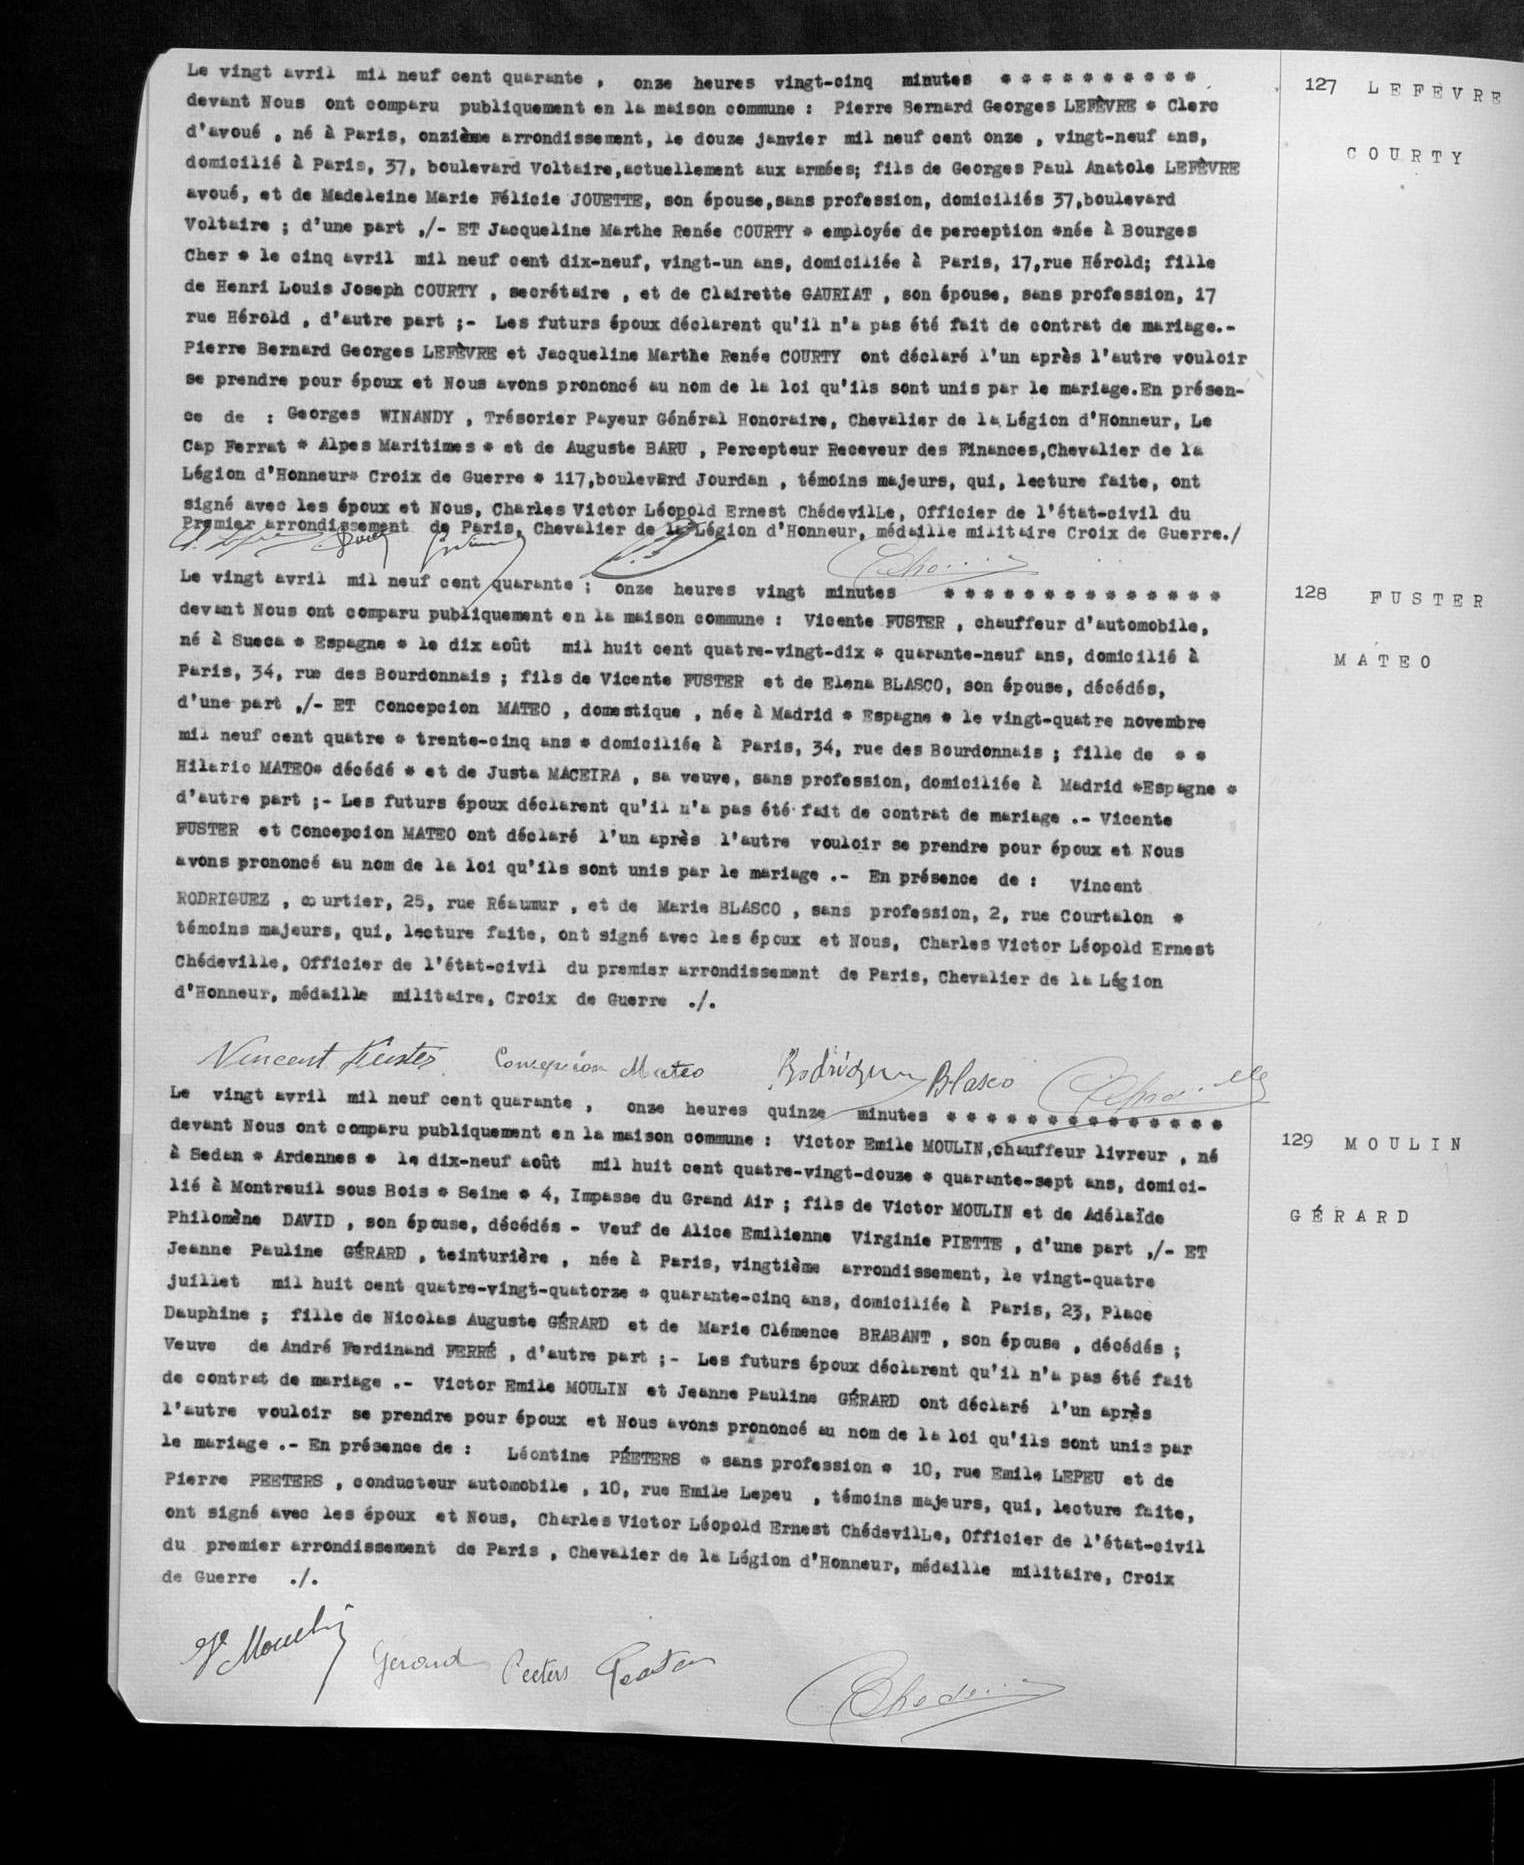Le vingt avril mil neuf cent quarante, onze heures vingt-cinq minutes ****
devant Nous ont comparu publiquement en la maison commune: Pierre Bernard George LEFEVRE * Clerc
d'avoué, né à Paris, onzième arrondissement, le douze janvier mil neuf cent onze, vingt-neuf ans,
domicilié à Paris, 37, boulevard Voltaire, actuellement aux armées; fils de Georges Paul Anatole LEFEVRE
avoué, et de Madeleine Marie Félicie JOUETTE, son épouse, sans profession, domiciliés 37, boulevard
Voltaire; d'une part ,/-ET Jacqueline Marthe Renée COURTY * employée de perception * née à Bourges
Cher * le cinq avril mil neuf cent dix-neuf, vingt-un ans, domiciliée à Paris, 17, rue Hérold; fille
de Henri Louis Joseph COURTY, secrétaire, et de Clairette GAURIAT, son épouse, sans profession, 17
rue Hérold, d'autre part ;-Les futurs époux déclarent qu'il n'a pas été fait de contrat de mariage.
Pierre Bernard Georges LEFEVRE et Jacqueline Marthe Renée COURTY ont déclaré l'un après l'autre vouloir
se prendre pour époux et Nous avons prononcé au nom de la loi qu'ils sont unis par le mariage. En présen-
ce de: Georges WINANDY, Trésorier Payeur Général Honoraire, Chevalier de la Légion d'Honneur, Le
Cap Ferrat * Alpes Maritimes * et de Auguste BARU, Pecepteur Receveur des Finances, Chevalier de la
Légion d'Honneur * Croix de Guerre * 117, boulevard Jourdan, témoins majeurs, qui, lecture faite, ont
signé avec les époux et Nous, Charles Victor Léopold Ernest Chédeville, Officier de l'état-civil du
Premier arrondissement de Paris, Chevalier de la Légion d'Honneur, médaille militaire Croix de Guerre ./
Le vingt avril mil neuf cent quarante; onze heures vingt minutes ****
devant Nous ont comparu publiquement en la maison commune: Vincente FUSTER, chauffeur d'automobile;
né à Sueca * Espagne * le dix août mil huit cent quatre-vingt-dix * quarante-neuf ans, domicilié à
Paris, 34, rue des Bourdonnais; fils de Vincente FUSTER et de Elena BLASCO, son épouse, décédés,
d'une part ,/-ET Concepcion MATEO, domestique, née à Madrid * Espagne * le vingt-quatre novembre
mil neuf cent quatre * trente-cinq ans * domiciliée à Paris, 34, rue des Bourdonnais; fille de **
Hilario MATEO * décédé * et de Juste MACEIRA, sa veuve, sans profession, domiciliée à Madrid *Espagne *
d'autre part ;-Les futurs époux déclarent qu'il n'a pas été fait de contrat de mariage .-Vincente
FUSTER et Conception MATEO ont déclaré l'un après l'autre vouloir se prendre pour époux et Nous
avons prononcé au nom de la loi qu'ils sont unis par le mariage .-En présence de: Vincent
RODRIGUEZ, courtier, 25, rue Réaumur, et de Marie BLASCO, sans profession, 2, rue Courtalon *
témoins majeurs, qui, lecture faite, ont signé avec les époux et Nous, Charles Victor Léopold Ernest
Chédeville, Officier de l'état-civil du premier arrondissement de Paris, Chevalier de la Légion
d'Honneur, médailler militaire, Croix de Guerre ./.
Le vingt avril mil neuf cent quarante, onze heures quinze minutes ****
devant Nous ont comparu publiquement en la maison commune: Victor Emile MOULIN, chauffeur livreur, né
à Sedan * Ardennes * le dix-neuf août mil huit cent quatre-vingt-douze * quarante-sept ans, domici-
lié à Montreuil sous Bois * Seine * 4, Impasse du Grand Air; fils de Victor MOULIN et de Adélaïde
Philomène DAVID, son épouse, décédés-Veuf de Alice Emilienne Virginie PIETTE, d'une part ,/-ET
Jeanne Pauline GERARD, teinturière, née à Paris, vingtième arrondissement, le vingt-quatre
juillet mil huit cent quatre-vingt-quatorze * quarante-cinq ans, domiciliée à Paris, 23, Place
Dauphine; fille de Nicolas Auguste GERARD et de Marie Clémence BRABANT, son épouse, décédés;
Veuve de André Ferdinand FERRE, d'autre part ;-Les futurs époux déclarent qu'il n'a pas été fait
de contrat de mariage .-Victor Emile MOULIN et Jeanne Pauline GERARD ont déclaré l'un après
l'autre vouloir se prendre pour époux et Nous avons prononcé au nom de la loi qu'ils sont unis par
le mariage .-En présence de: Léontine PEETERS * sans profession * 10, rue Emile LEPEU et de
Pierre PEETERS, conducteur automobile, 10, rue Emile Lepeu, témoins majeurs, qui, lecture faite,
ont signé avec les époux et Nous, Charles Victor Léopold Ernest Chédeville, Officier de l'état-civil
du premier arrondissement de Paris, Chevalier de la Légion d'Honneur, médaille militaire, Croix
de Guerre ./.
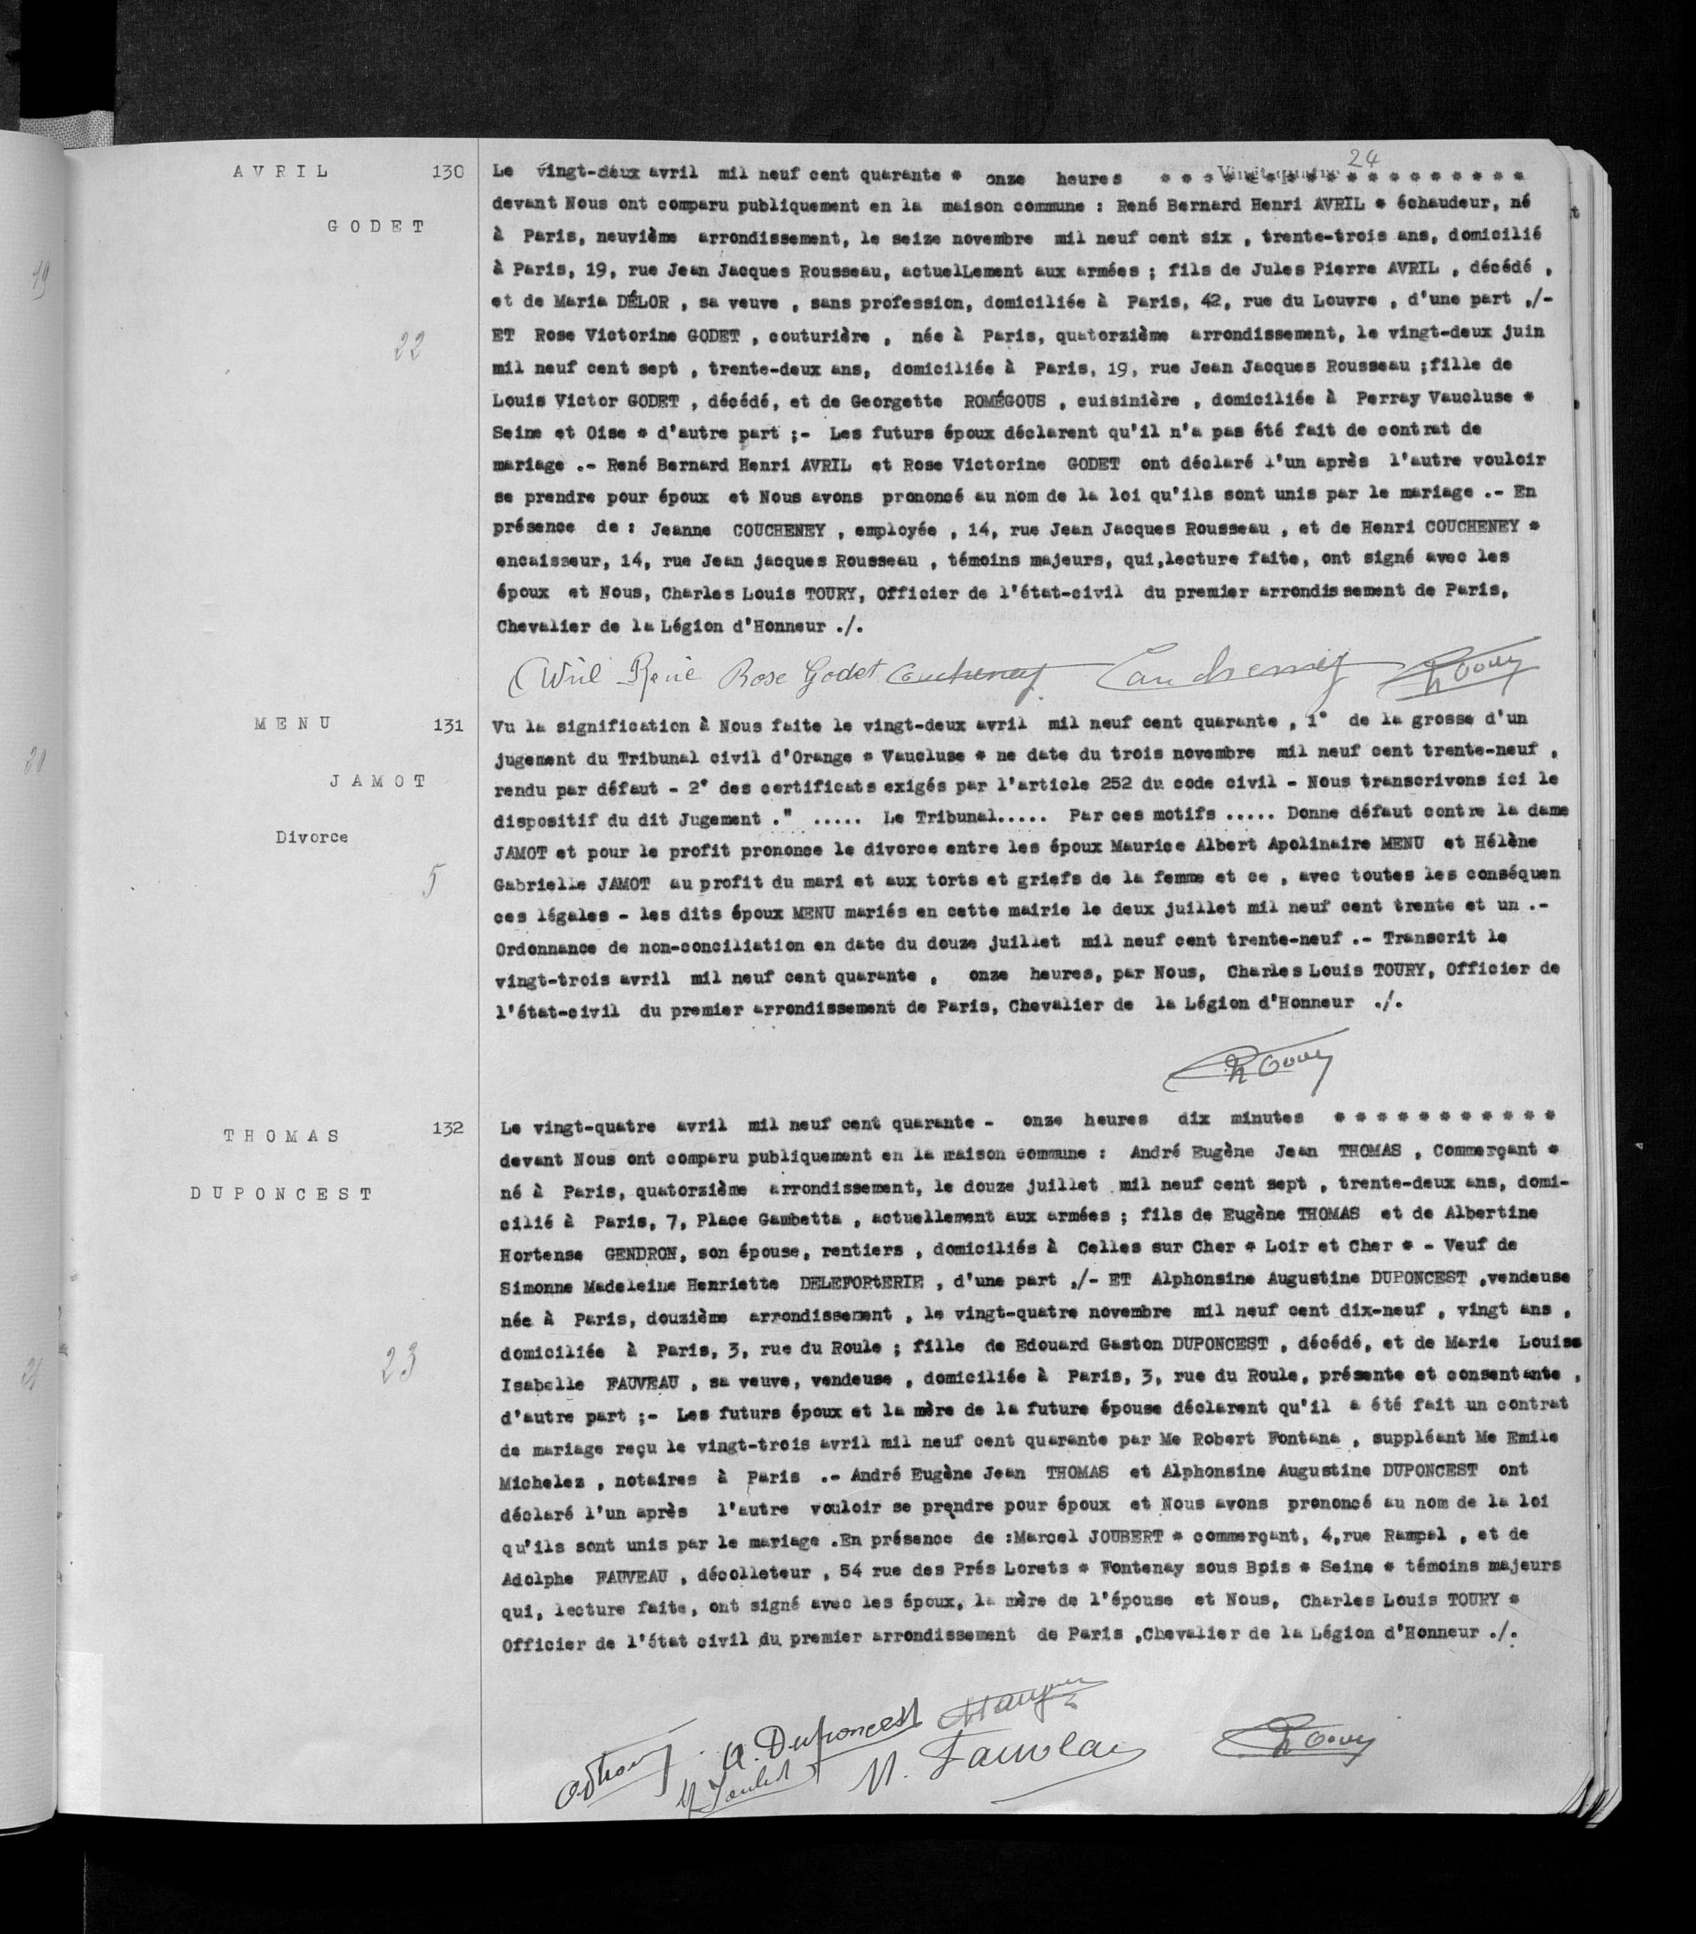Le vingt-deux avril mil neuf cent quarante * onze heures ****
devant Nous ont comparu publiquement en la maison commune: René Bernard Henri AVRIL * échangeur, né
à Paris, neuvième arrondissement, le seize novembre mil neuf cent six, trente-trois ans, domicilié
à Paris, 19, rue Jean Jacques Rousseau, actuellement aux armées; fils de Jules Pierre AVRiL, décédé,
et de Maria DECLOR, sa veuve, sans profession, domiciliée à Paris, 42, rue de Louvre, d'une part ,/
ET Rose Victorine GODET, couturière, née à Paris, quatorzième arrondissement, le vingt-deux juin
mil neuf cent sept, trente-deux ans, domiciliée à Paris, 19, rue Jean Jacques Rousseau; fille de
Louis Victor GODET, décédé, et de Georgette ROMEGOUS, cuisinière, domiciliée à Perray Vaucluse *
Seine et Oise * d'autre part ;-Les futurs époux déclarent qu'il n'a pas été fait de contrat de
mariage .-René Bernard Henri AVRIL et Rose Victorine GODET ont déclaré l'un après l'autre vouloir
se prendre pour époux et Nous avons prononcé au nom de la loi qu'ils sont unis par le mariage .-En
présence de: Jeanne COUCHENEY, employée, 14, rue Jean Jacques Rousseau, et de Henri COUCHENEY *
encaisseur, 14, rue Jean jacques Rousseau, témoins majeurs, qui, lecture faite, ont signé avec les
époux et Nous, Charles Louis TOURY, Officier de l'état-civil du premier arrondissement de Paris,
Chevalier de la Légion d'Honneur ./.
Vu la signification à Nous faite le vingt-deux avril mil neuf cent quarante, 1° de la grosse d'un
jugement du Tribunal civil d'Orange * Vaucluse * ne date du trois novembre mil neuf cent trente-neuf,
rendu par défaut-2° des certificats exigés par l'article 252 du code civil-Nous transcrivons ici le
dispositif du dit Jugement." ..... Le Tribunal..... Par ces motifs..... Donne défaut contre la dame
JAMOT et pour le profit prononce le divorce entre les époux Maurice Albert Apolinaire MENU et Hélène
Gabrielle JAMOT au profit du mari et aux torts et griefs de la femme et ce, avec toutes les conséquen
ces légales-les dits époux MENU mariés en cette mairie le deux juillet mil neuf cent trente et un.
Ordonnance de non-conciliation en date du douze juillet mil neuf cent trente-neuf .-Transcrit le
vingt-trois avril mil neuf cent quarante, onze heures, par nous, Charles Louis TOURY, Officier de
l'état-civil du premier arrondissement de Paris, Chevalier de la Légion d'Honneur ./.
Le vingt-quatre avril mil neuf cent quarante-onze heures dix minutes ****
devant Nous ont comparu publiquement en la maison commune: André Eugène Jean THOMAS, Commerçant *
né à Paris, quatorzième arrondissement, le douze juillet mil neuf cent sept, trente-deux ans, domici-
lié à Paris, 7, Place Gambetta, actuellement aux armées; fils de Eugène THOMAS et de Albertine
Hortense GENDRON, son épouse, rentiers, domiciliés à Celles sur Cher * Loir et Cher *-Veuf de
Simonne Madeleine Henriette DELEFORtERiE, d'une part ,/-ET Alphonsine Augustine DUPONCEST, vendeuse
née à Paris, douzième arrondissement, le vingt-quatre novembre mil neuf cent dix-neuf, vingt ans,
domiciliée à Paris, 3, rue du Roule; fille de Edouard Gaston DUPONCEST, décédé, et de Marie Louise
Isabelle FAUVEAU, sa veuve, vendeuse, domiciliée à Paris, 3, rue du Roule, présente et consentante,
d'autre part ;-Les futurs époux et la mère de la future épouse déclarent qu'il a été fait un contrat
de mariage reçu le vingt-trois avril mil neuf cent quarante par Me Robert Fontana, suppléant Me Emile
Michelez, notaires à Paris .-André Eugène Jean THOMAS et Alphonsine Augustine DUPONCEST ont
déclaré l'un après l'autre vouloir se prendre pour époux et Nous avons prononcé au nom de la loi
qu'ils sont unis par le mariage. En présence de: Marcel JOUBERT * commerçant, 4, rue Rampal, et de
Adolphe FAUVEAU, décolleteur, 54 rue des Prés Lorets * Fontenay sous Bois * Seine * témoins majeurs
qui, lecture faite, ont signé avec les époux, la mère de l'épouse et Nous, Charles Louis TOURY *
Officier de l'état civil du premier arrondissement de Paris, Chevalier de la Légion d'Honneur ./.